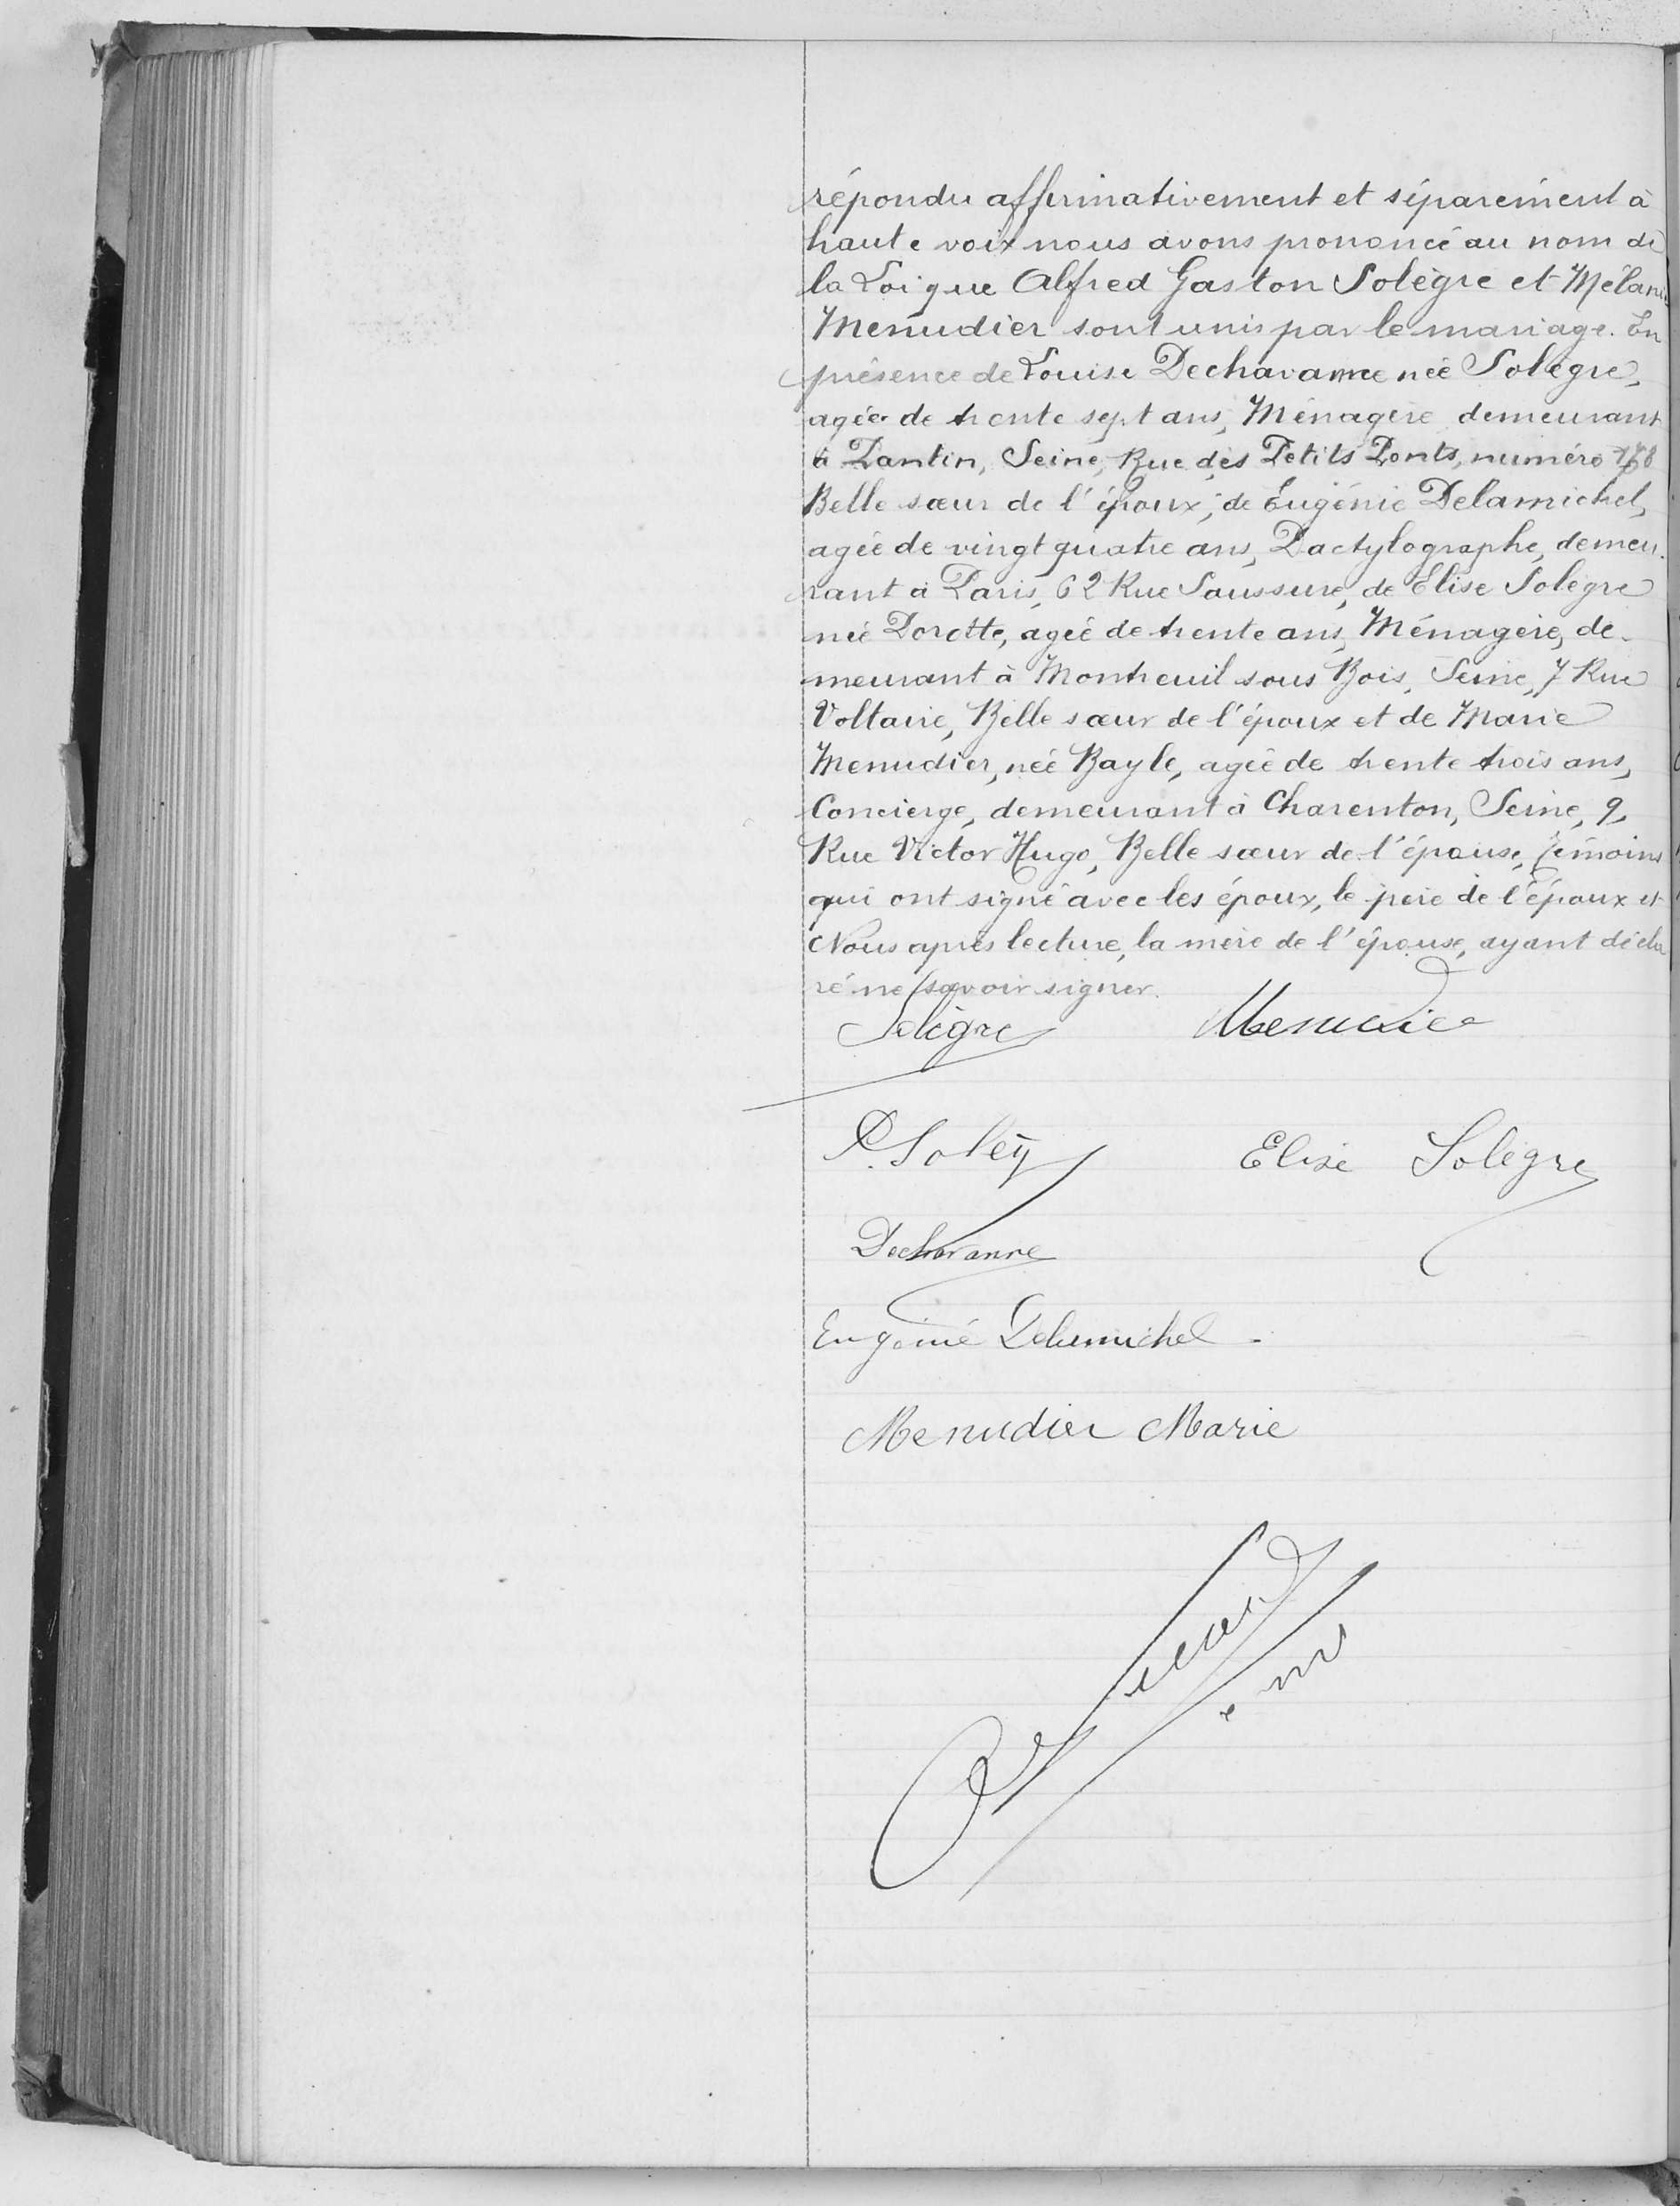répondu affirmativement et séparément à
haute voix nous avons prononcé au nom de
la Loi que Alfred Gaston Solègre et Melanie
Menudier sont unis par le mariage. En
présence de Louis Dechavame née Solègre
agée de trente sept ans, Ménagère, demeurant
à Pantin, Seine, Rue des Petits Ponts, numéro 168
Belle soeur de l'époux, de Eugénie Delamichel,
agée de vingt quatre ans, Dactylographe, demeu
rant à Paris, 62 Rue Saussure, de Elise Solègre
née Dorette, agée de trente ans, Ménagère, de-
meurant à Montreuil sous Bois, Seine, 7 Rue
Voltaire, Belle soeur de l'époux et de Marie
Menudier, née Bayle, agée de trente trois ans,
Concierge, demeurant à Charenton, Seine, 9,
Rue Victor Hugo, Belle soeur de l'épouse, témoins
qui ont signé avec les époux, le père de l'époux et
Nous après lecture, la mère de l'épouse, ayant décla
ré ne savoir signer
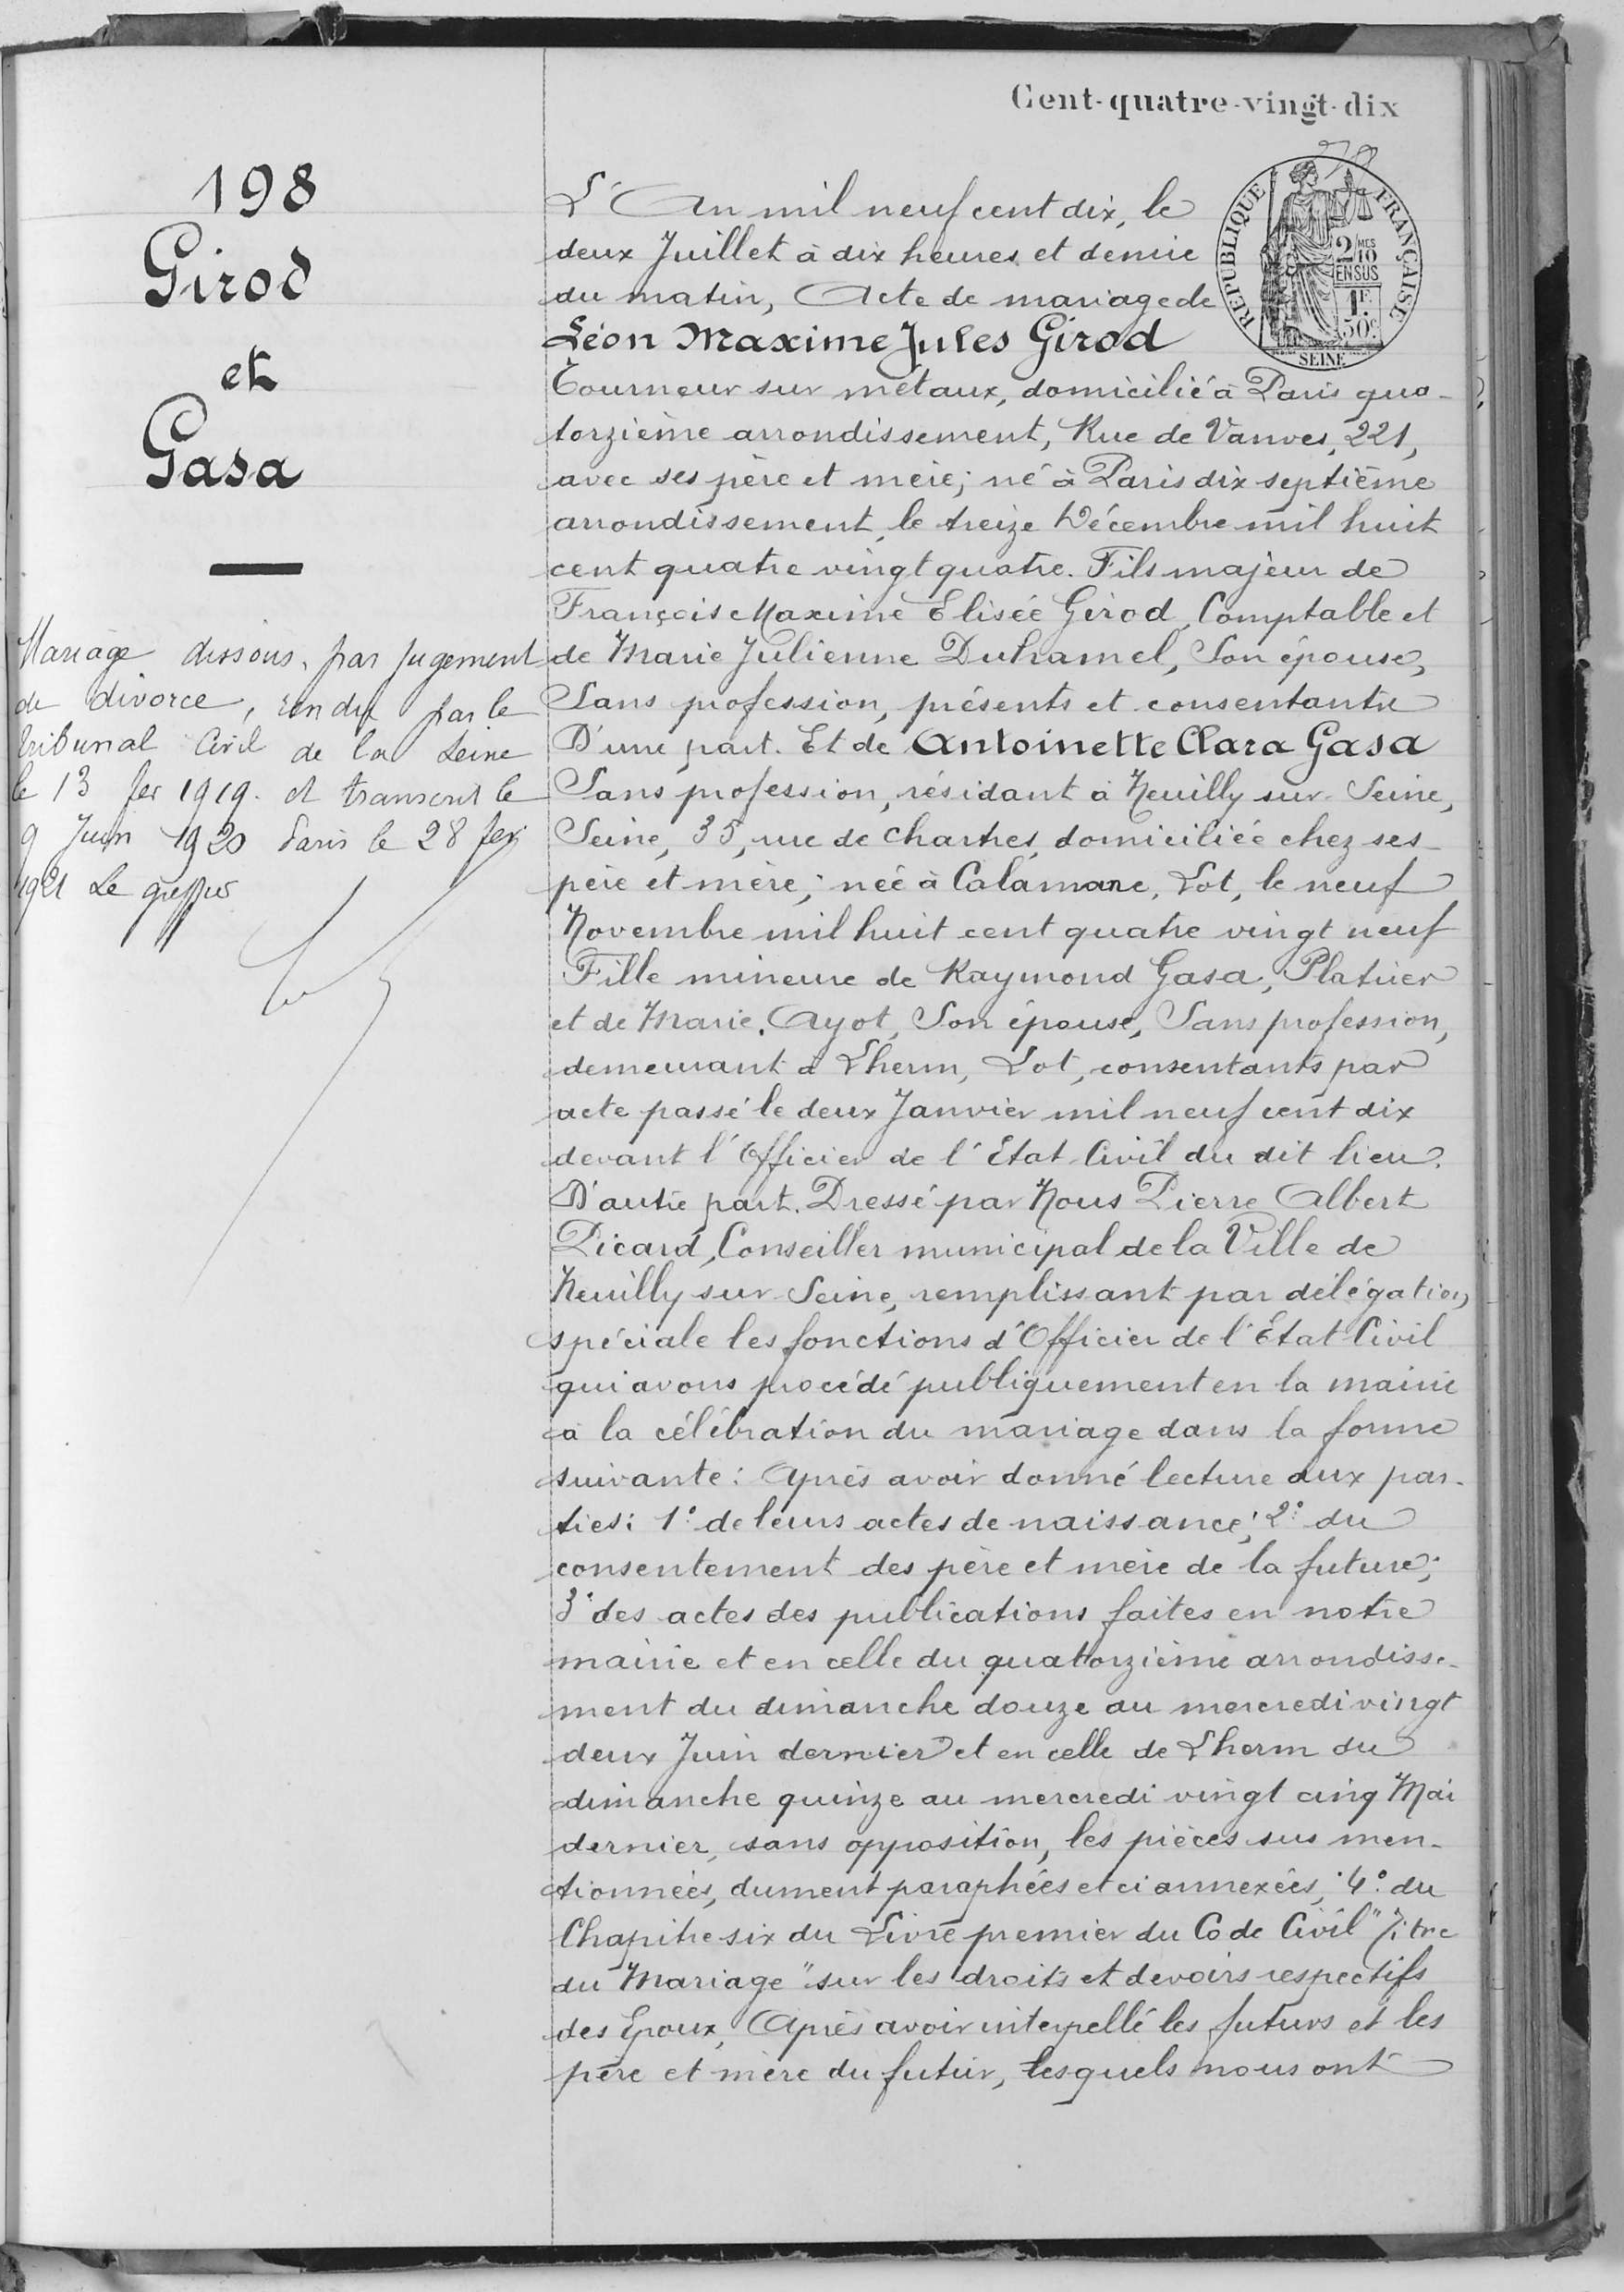198
Girod
et
Pasa
L'An mil neuf cent dix, le
deux Juillet à dix heures et demie
du matin, Acte de mariage de
Léon Maxime Jules Girod
Tourneur sur métaux, domicilié à Paris qua-
torzième arrondissement, Rue de Vanves, 221
avec ses père et mère; né à Paris dix septième
arrondissement le treize Décembre mil huit
cent quatre vingt quatre. Fils majeur de
Françoise Maxime Elisée Girod Comptable et
de Marie Julienne Duhamel, Son épouse
Sans profession, présents et consentante
D'une part. Et de Antoinette Clara Gasa
Sans profession, résidant à Neuilly sur Seine,
Seine, 35, rue de Chartres, domiciliée chez ses
Père et mère, née à Calamone, Lot, le neuf
Novembre mil huit cent quatre vingt neuf
Fille mineure de Raymond Gasa, Platier
et de Marie Cayot Son épouse, Sans profession,
demeurant à Therm, Lot, consentant par
acte passé le deux Janvier mil neuf cent dix
devant l'Officier de l'état Civil du dit lieu.
D'autre part. Dressé par Nous Pierre Albert
Picard, Conseille municipal de la Ville de
Neuilly sur Seine, remplissant par délégation
spéciale les fonctions d'Officier de l'Etat Civil
qui avons procédé publiquement en la mairie
à la célébration du mariage dans la forme
suivante: Après avoir donné lecture aux par-
ties: 1° de leurs actes de naissance; 2° du
consentement des père et mère de la future
3° des actes des publications faites en notre
mairie et en celle du quatorzième arrondisse-
ment du dimanche douze au mercredi vingt
deux Juin dernier et en celle de Thorm du 3
dimanche quinze au mercredi vingt cinq Mai
dernier, sans opposition, les pièces sus men-
tionnées, dument paraphées et ci annexées; 4° du
Chapitre six du Livre premier du Code Civil "Titre
du Mariage" sur les droits et devoirs respectifs
des Epoux, Après avoir interpellé les futurs et les
père et mère du futur, lesquels nous ont